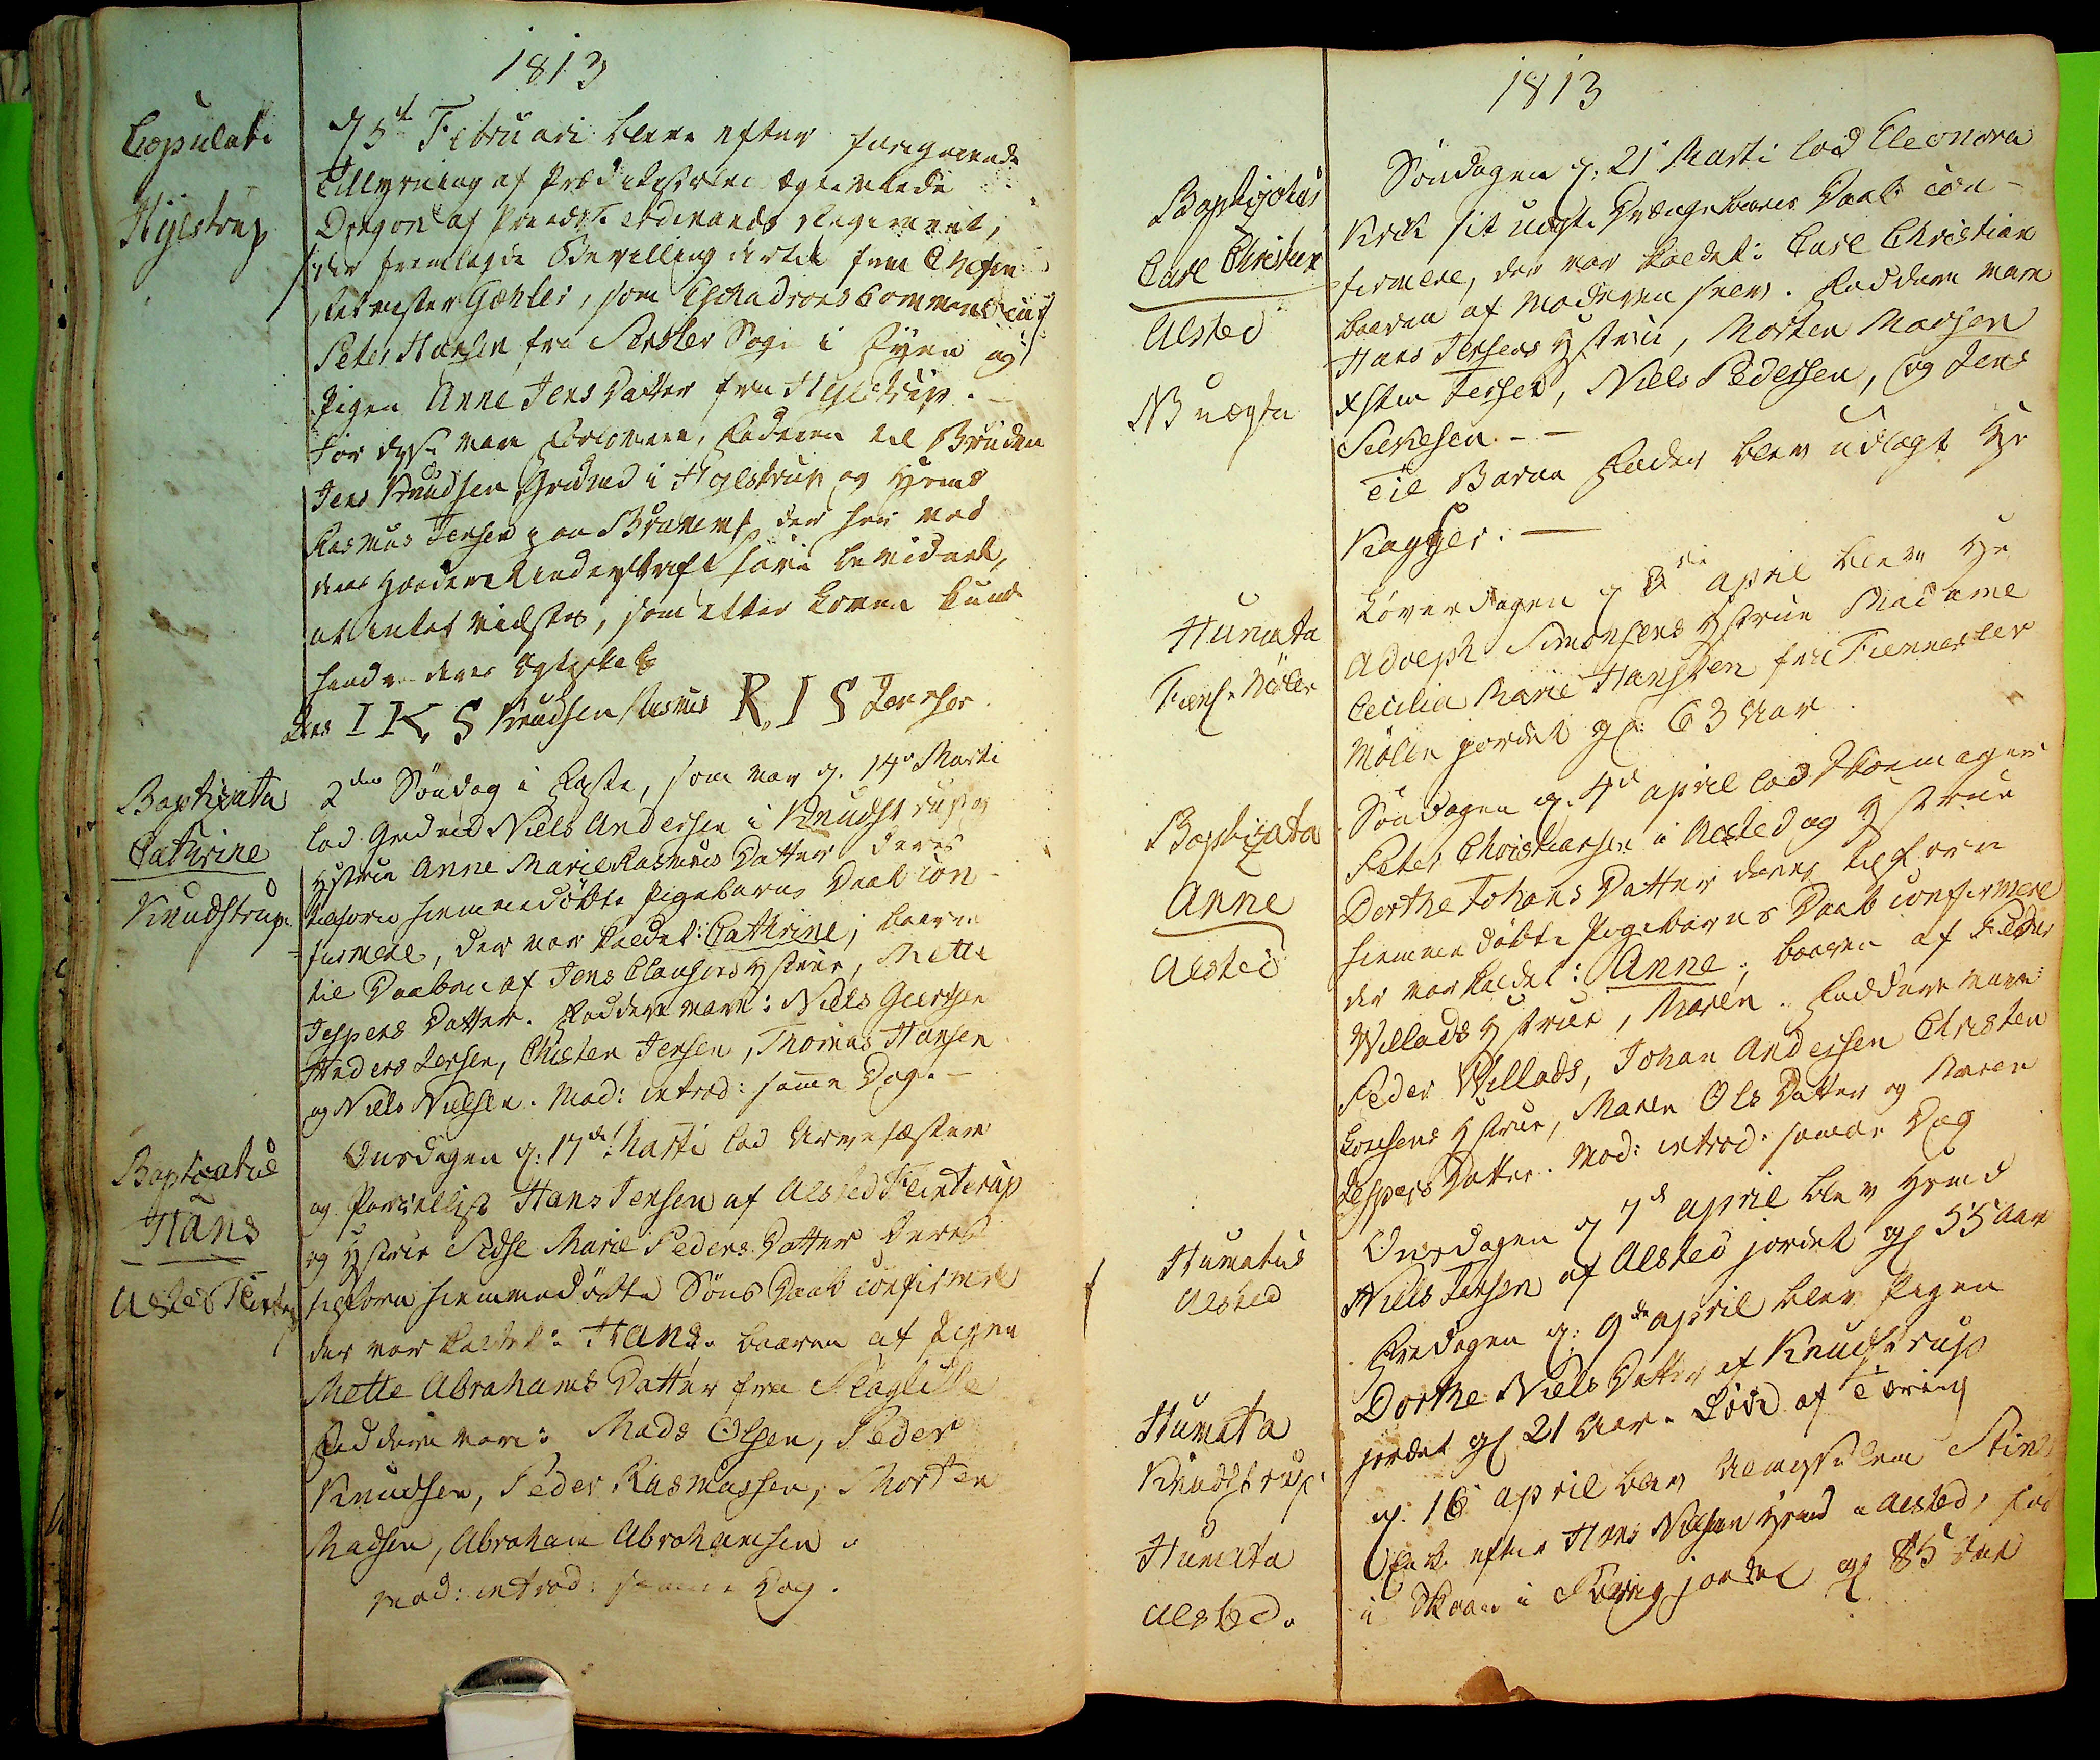Copulati
Hylstrup
Baptizata
Cathrine
Kundstrup
Baptizatus
Hans
Alsted Flinterup
1813
d 5te Februari bleve efter foregaaende
Tillysning af Prædisen ægteviede
Dragon af Prinds Ferdinands Regiment,
efter fremlagde Bevilling dertil fra Chefen
Ritmester Gæhle, som Eschadrons Com##
Peder Hansen fra Serslev Sogn i Fyen og
Pigen Anne Jens Datter fra Hylstrup.
for disse vare Forlovere, Faderen til Bruden
Jens Knudsen Grdmd i Hylstrup og Hsmd
Rasmus Jensen paa Brunem## der her ved
deres Hænders Underskrift have bevidnet,
at intet vidstes, som efter Loven kunde
hindre deres Ægteskab
Jens I K S Knudsen Rasmus R I S Jensen
2den Søndag i Faste, som var d: 14de Marti
lod Grdmd Niels Anderssen i Knudstrup og
Hstrue Anne Marie Rathans Datter deres
tilforn hiemmedøbte Pigebarns Daab con¬
firmere, der var kaldet: Cathrine; baaren
til Daaben af Jens Clausens Hstrue, Mette
Jespers Datter. Faddere vare: Niels Geertsen
Anders Jensen, Christen Jensen, Thomas Hansen
og Niels Nielsen. Mod: introd: samme Dag.
Onsdagen d: 17de Marti lod Arvefæster
og Parcellist Hans Jensen af Alsted Flinterup
og Hstrue Sidse Marie Peders Datter deres
tilforn hiemmedøbte Søns Daab confirmere
der var kaldet: Hans. baaren af Pigen
Mette Abrahams Datter fra Slaglille
Fadderne vare: Mads Olsen, Peder
Knudsen, Peder Rasmussen, Morten
Madsen, Abraham Abrahamsen
Mod: introd: samme Dag.
Baptizatus
Carl Christen
Alsted
NB uægte
Humata
Fiens. Mølle
Baptizata
Anne
Alsted
Hamatus.
Alsted
Humata
Knudstrup
Humata
Alsted.
1813
Søndagen d: 21 Marti lod Eleonora
K## sit uægte Drengebarns Daab con¬
firmere, der var kaldet: Carl Christian
baaren af Moderen slev. Faddere vare
Hans Jensens Hustru, Morten Madsen
Xsten Je##sen, Niels Pedersen, og Jens
Silkesen.
Til Barne Fader blev udlagt Hr
Kayser
Løverdagen d 3die April blev Hr
Adolph Simensens Hstrue Madame
Cecilia Marie Hansen Fienneslev
Mølle jordet gl: 63 Aar.
Søndagen d: 4de April lod Skoemager
Peter Christiansen i Alsted og Hstrue
Dorthe Johans Datter deres tilforn
hiemmedøbte Pigebarns Daab confirmere
der var kaldet: Anne; baaren af Peder
Willads Hstrue, Maren. Faddere vare:
Peder Willads, Johan Anderesen Christen
Lousens Hstrue, Maren Ols Datter og Maren 
Jespers Datter. Mod: introd: samme Dag
Onsdagen d 7de April blev Hsmd
Niels Jensen af Alsted jordet gl 55 Aar
Fredagen d: 9de April blev Pigen
Dorthe Niels Datter af Knudstrup
jordet gl 21 Aar. døde af Tæring
d: 16 April blev Almisselem Stine
Enke efter Hans Nielsen Hsmd i Alsted, fød
i Skaane i Sverrig jordet gl 85 Aar
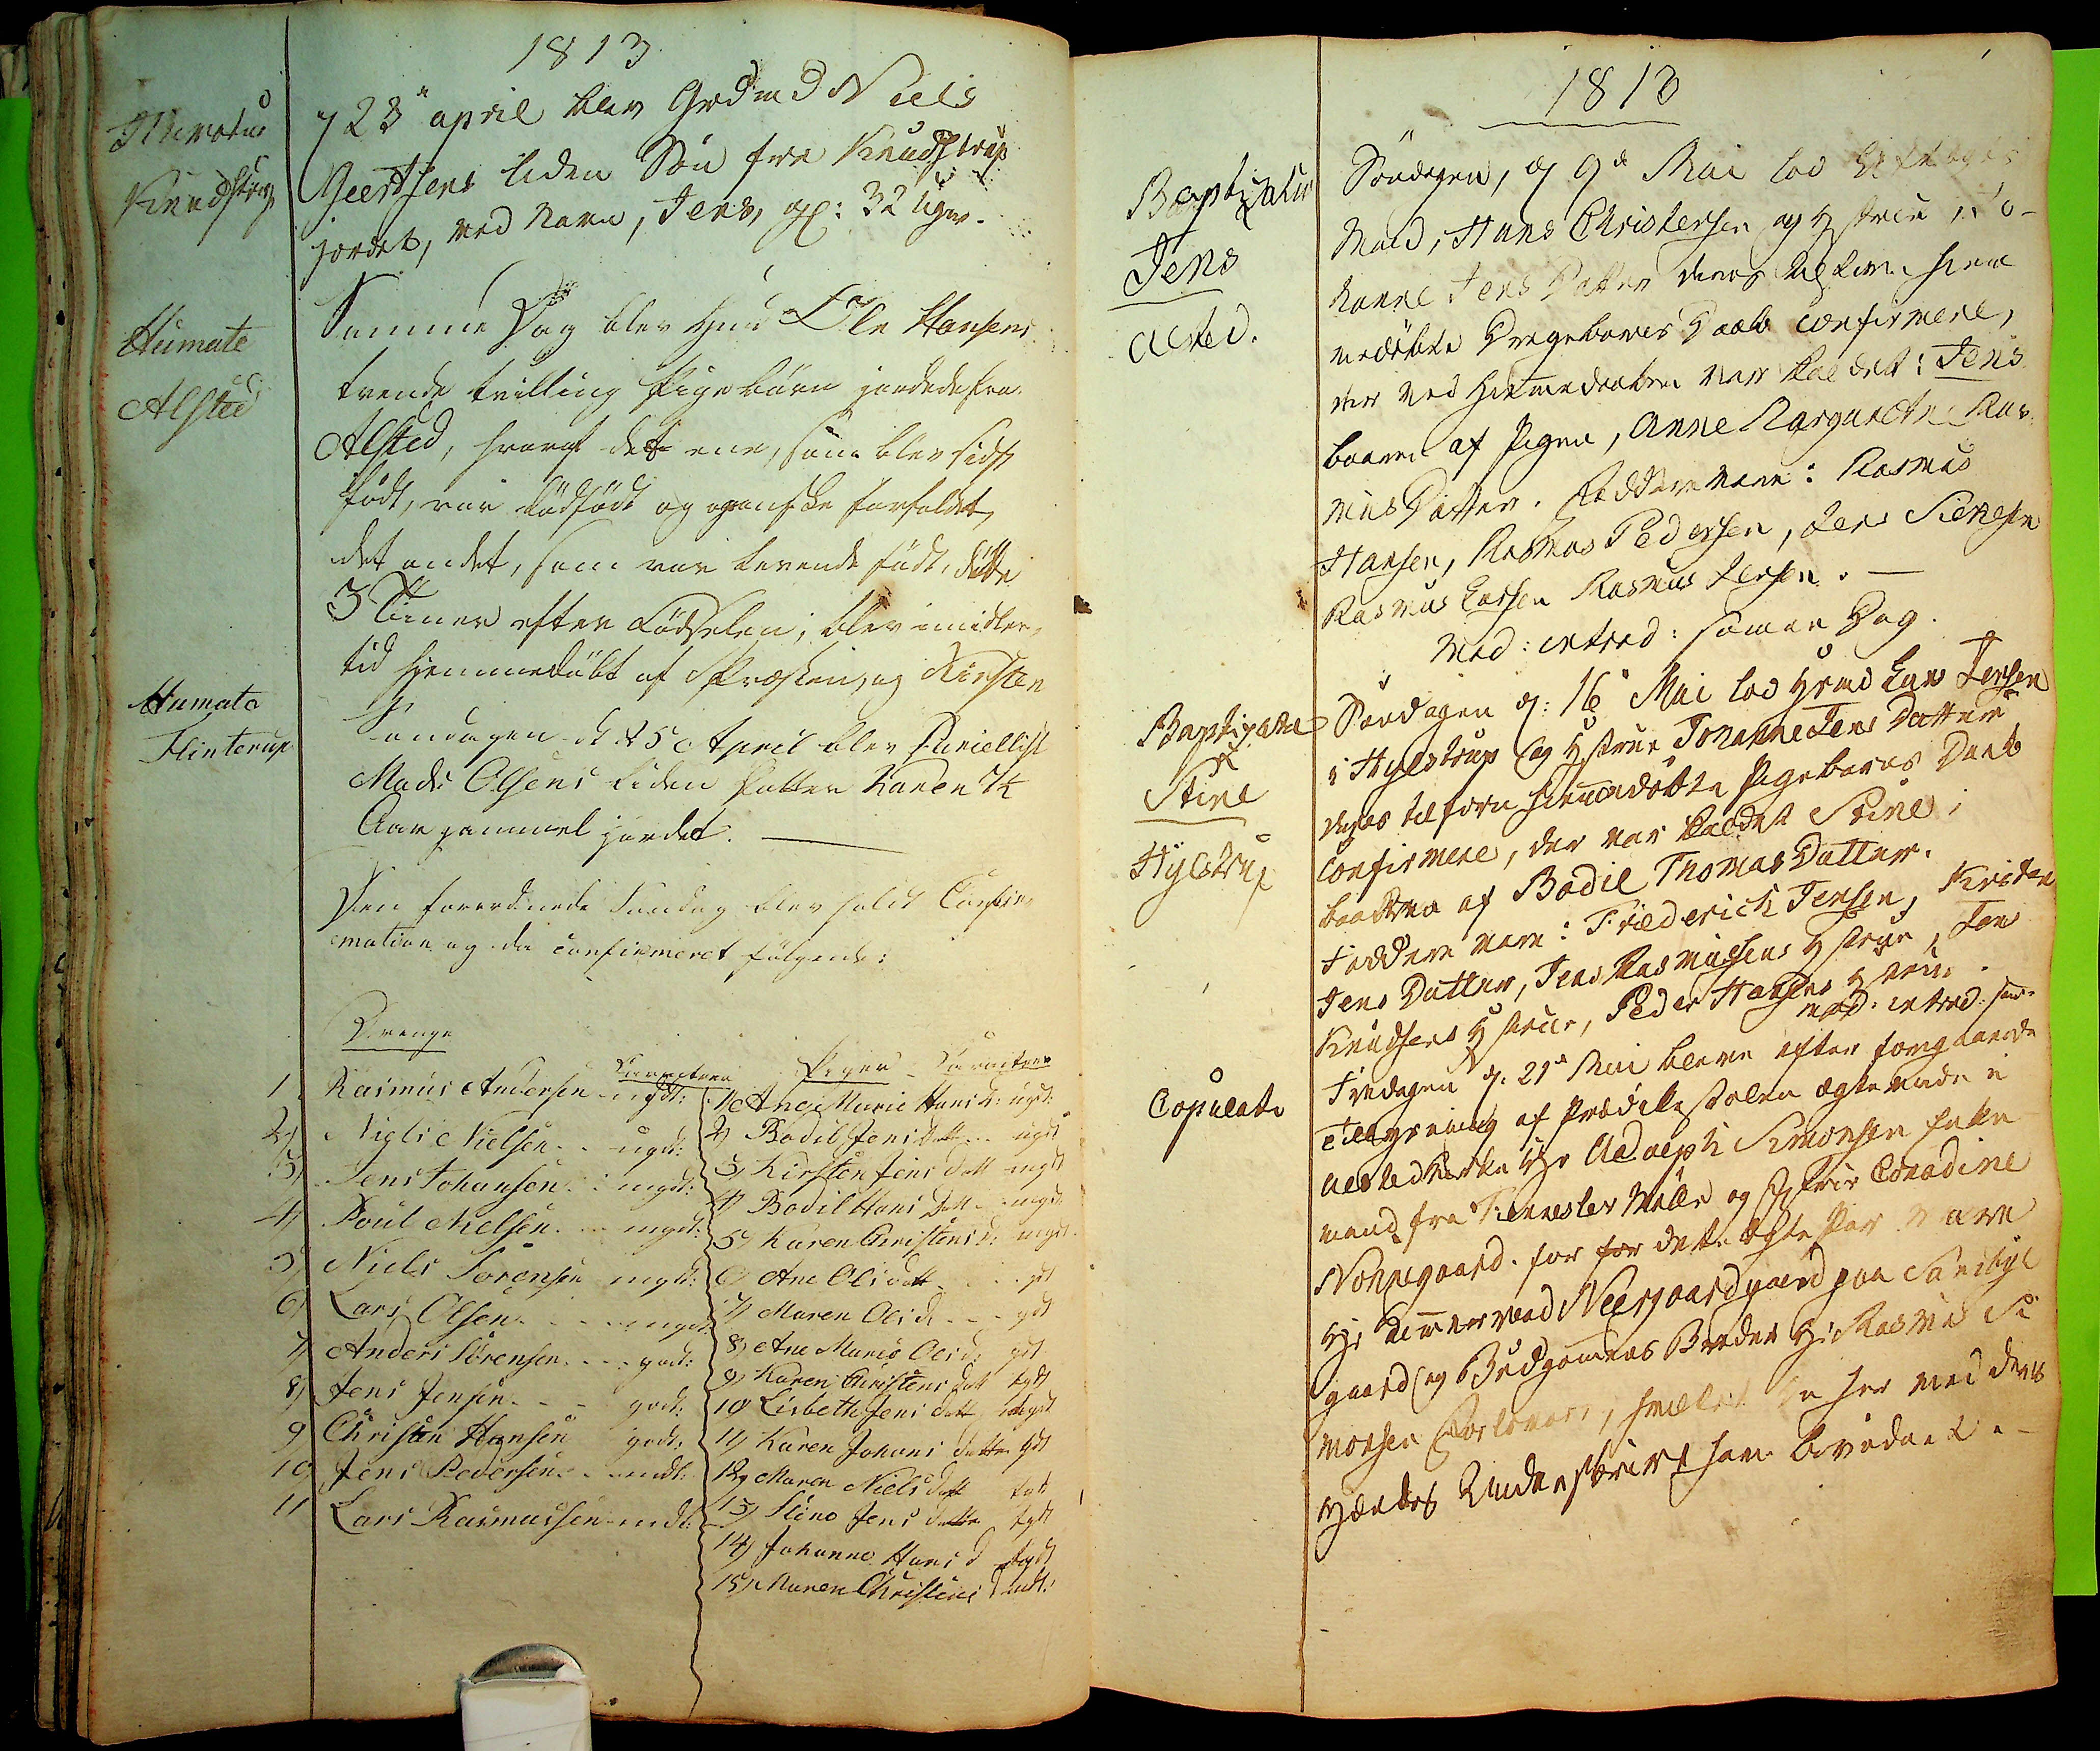Humatus
Knudstrup
Humata
Alsted
Humata
Flinterup
1813
d 28 April blev Grdmd Niels
Geertsens liden Søn fra Knudstrup
jordet ved Navn, Jens, gl: 32 Uger.
Samme Dag blev Hmd Ole Hansens
tvende tvilling Pigebørn jordedede fra
Alsted, hvoraf det ene, som blev sidst
født, var dødfødt og ##anske forholdt
det andet, som var levende født, døde
3 Timer efter Fødselen, blev imidler
tid hjemmedøbt af Præsten og Kirsten
Søndagen d 25 April blev Parcellist
Mads Olsens liden Datter Karen 7½
Aar gammel jordet.
Den forerdiende Søndag blev holdt Confir
mation og da confirmeret følgende:
Drenge
Karaqtren
1 Rasmus Andersen - ugdt:
2 Niels Nielsen - - ugdt:
3 Jens Johansen - - mgdt:
4 Poul Nielsen - - - mgdt:
5 Niels Sørensen mgdt:
6 Lars Olsen - - - mgdt:
7 Anders Sørensen - - - godt:
8 Jens Jensen - - - godt:
9 Christen Hansen - - - godt:
10 Jens Pedersen - mdl:
11 Lars Rasmussen - mdl
Piger Karaqtren
1 Ane Marie Hans D: ugdt:
2 Bodil Jens Datter - - ugdt:
3 Kirsten Jens Datt mgdt:
4 Bodil Hans Datt mgdt:
5 Karen Christens D: mgdt:
6 Ane Ols Datt - - - gdt
7 Maren Ols D: - - -  gdt
8 Ane Marie Ols D: gdt
9 Karen Christens Datt tgdt
10 Lisbeth Jens Datt tgdt
11 Karen Johans Datter tgdt
12 Maren Niels Datt tgdtt
13 Stine Jens Datt tgdt
14 Johanne Hans D tgdt
15 Maren Christens d mdl.
Baptizatus
Jens
Alsted.
Baptizata
Stine
Hylstrup
Copulati
1813
Søndagen, d 9de Mai lod Aftægts
Mand, Hans Christensen og Hstrue Jo¬
hanne Jens Datter derestilforn hiem
medøbte Drengebarns Daab confirmere,
der ved Hiemmedaaben var kaldet: Jens
baaren af Pigen Anne Margareth Ras
mus Datter. Faddere vare: Rasmus
Hansen, Rasmus Pedersen, Jens Silkesen
Rasmus Larsen Rasmus Jensen.
Mod: introd: samme Dag.
Søndagen d: 16 Mai lod Hsmd Lars Jensen
i Hylstrup og Hstrue Johanne Jens Datter
deres tilforn hiemmedøbte Pigebarns Daab
confirme, der var kaldet Stine;
baaren af Bodil Thomasdatter.
Faddere vare: Fræderich Jensen, Kirsten
Jens Datter, Jens Rasmusens Hstrue, Jens
Knudsens Hstrue, Peder Hansens Hstrue
Mod: introd: sa##
Fredagen d: 27de Mai bleve efter foregaaende
Tillydning af Prædikestolen ægteviede i
Alsted Kirke Hr Adolph Simonsens Enke
mand fra Fienneslev Mølle og Jfrue Coradine
Nonnegaard, for for dette  Ægte Par vare
Hr Kammerraad Neergaard gaard paa Sandbye
sgaard og Brudgommens Broder Hr Rasmus Si
monsen Forlovere, hvilket de her med deres
Hænders Underskrivt have bevidnet.